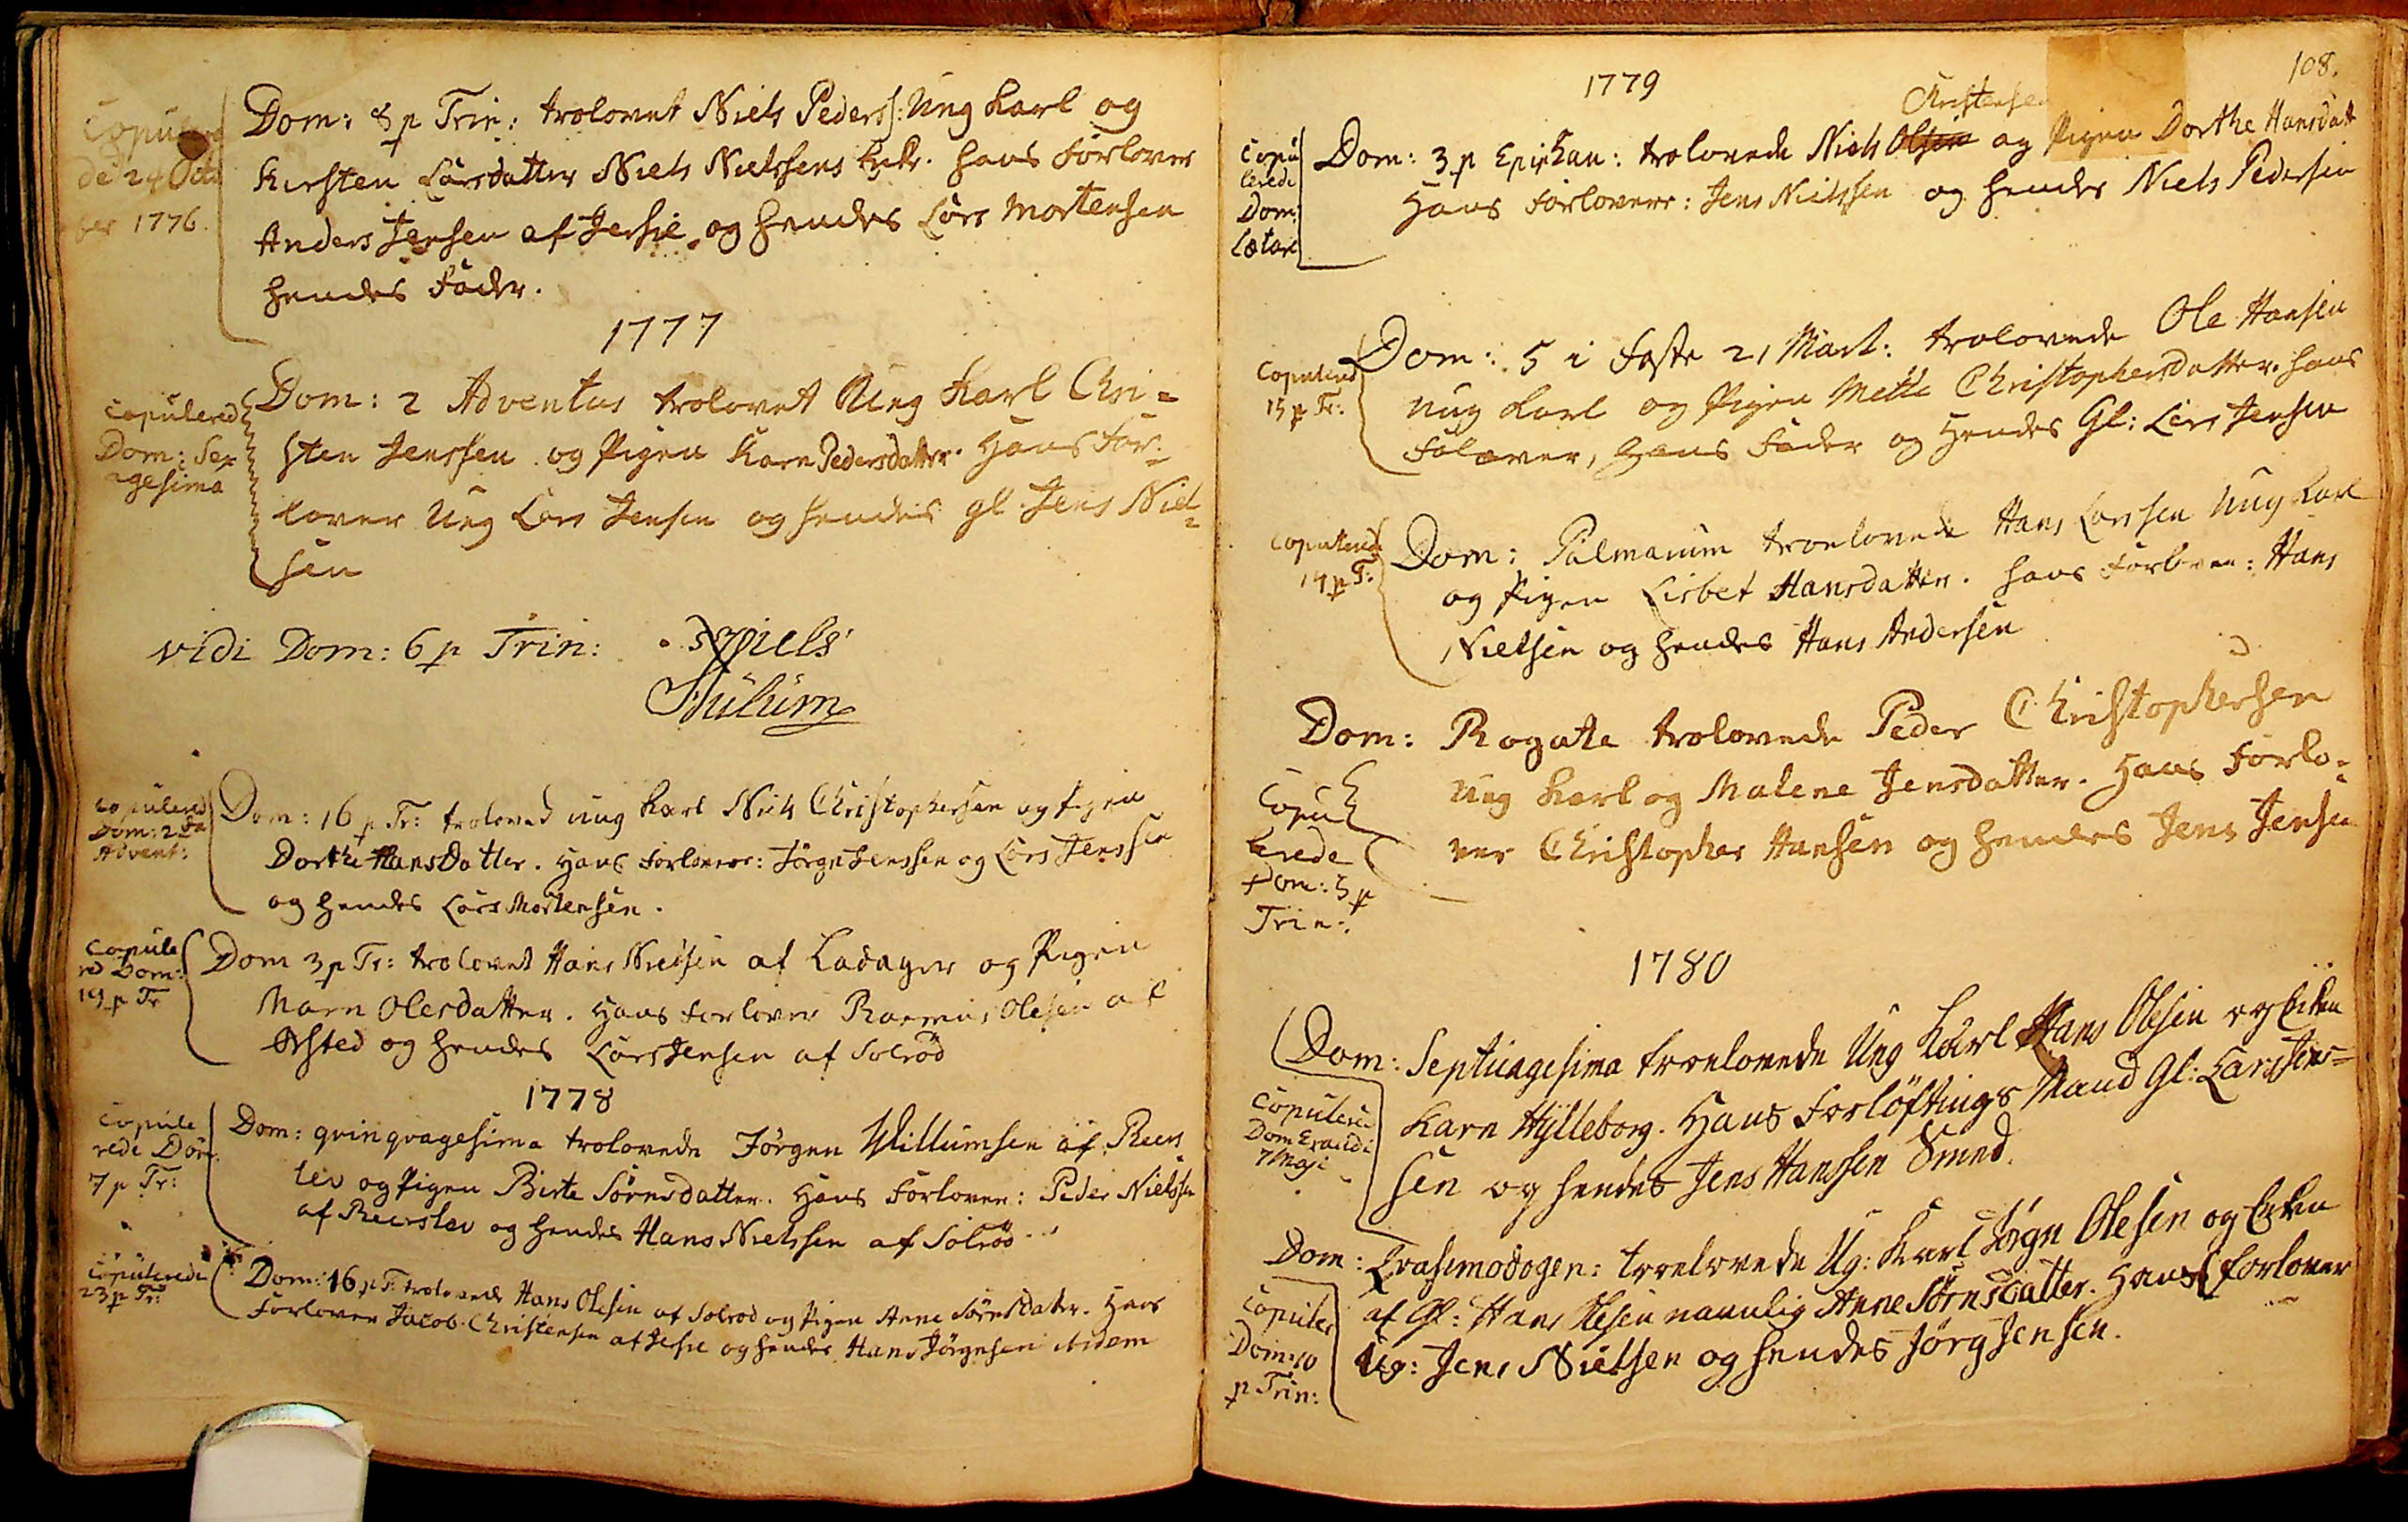Dom: 8 p Trin: trolovet Niels Pederss: Ung karl og
Kirsten Larsdatter Niels Nielsens Enke. hans Forlover
Anders Jensen af Jersie og hendes Lars Mortensen
hendes Fader.
Copulere
de 24 Octo
ber 1776.  
1777
Dom: 2 Adventus trolovet Ung karl Chri¬
sten Jenssen og Pigen Karn Pedersdatter. Hans For¬
lover Ung Lars Jensen og hendes gl. Jens Niel¬
sen
Copulered
Dom: Sex
agesima
Vidi Dom: 6 p Trin: Niels
Aulum
Dom: 16 p Tr: trolovet ung karl Niels Christophersen og Pigen
Dorthe HansDatter. Hans Forlovere: Jørgn Jenssen og Lars Jenssen
og hendes Lars Mortensen.
Copulered
Dom: 2da
Advent:
Dom 3 p tr: trolovet Hans Nielsen af Ladager og Pigen
Marn Olesdatter. Hans Forlover Rasmus Olesen af
Ørsted og hendes Lars Jensen af Solrød
Copule
red Dom:
19 p Tr.
1778
Dom: qvinqvagesima trolovede Jørgen Willumsen af Reers¬
lev og Pigen Birte Sørnsdatter. Hans Forlover: Peder Nielssen
af Reerslev og hendes Hans Nielssen af Solrød
Copule
rede Dom.
7 p Tr:      
Dom: 16 p T: trolovede Hans Olesen af Solrød og Pigen Anne Sørnsdatter. Hans
Forlover Jacob Christensen af Jersie og hendes Hans Jørnsen ibidem
Copulerede
23 p Tr:        
108.
1779
Christensen
Dom: 3 p Epiphan: trolovede Niels Olsen og Pigen Dorthe Hansdatt
Hans Forlovere: Jens Nielssen og hendes Niels Pedersen
Copu
lerede
Dom:
Lætar
Dom: 5 i Faste 21 Mart: trolovede Ole Hansen
ung karl og Pigen Mette Christophersdatter. hans
Forlover, hans Fader og Hendes Gl: Lars Jensen
Copulered
15 p Tr:
Dom: Palmarum troelovede Hans Larssen Ungkarl
og Pigen Lisbet Hansdatter. hans Forlover: Hans
Nielsen og hendes Hans Andersen
Copulered
14 p T:
Dom: Rogate trolovede Peder Christophersen
Ung karl og Malene Jensdatter. Hans Forlo¬
var Christopher Hansen og hendes Jens Jensen
Copu
lerede
Dom: 5 p
Trin:
1780
Dom: Septuagesima troelovede Ung Karl Hans Olesen og Enken
Karn Hylleborg. Hans Forløftings Mand Gl: Lars Jens¬
sen og hendes Jens Hanssen Smed.
Copulered
Dom Exaudi
7 Maji
Dom: Qvasimodogen: troelovede Un: Karl Jørgn Olesen og Enken
af Gl: Hans Olesen navnlig Anne Sørnsdatter. Hans Forlover
Ug: Jens Nielsen og hendes Jørg Jensen.
Copuler
Dom: 10
p Trin:
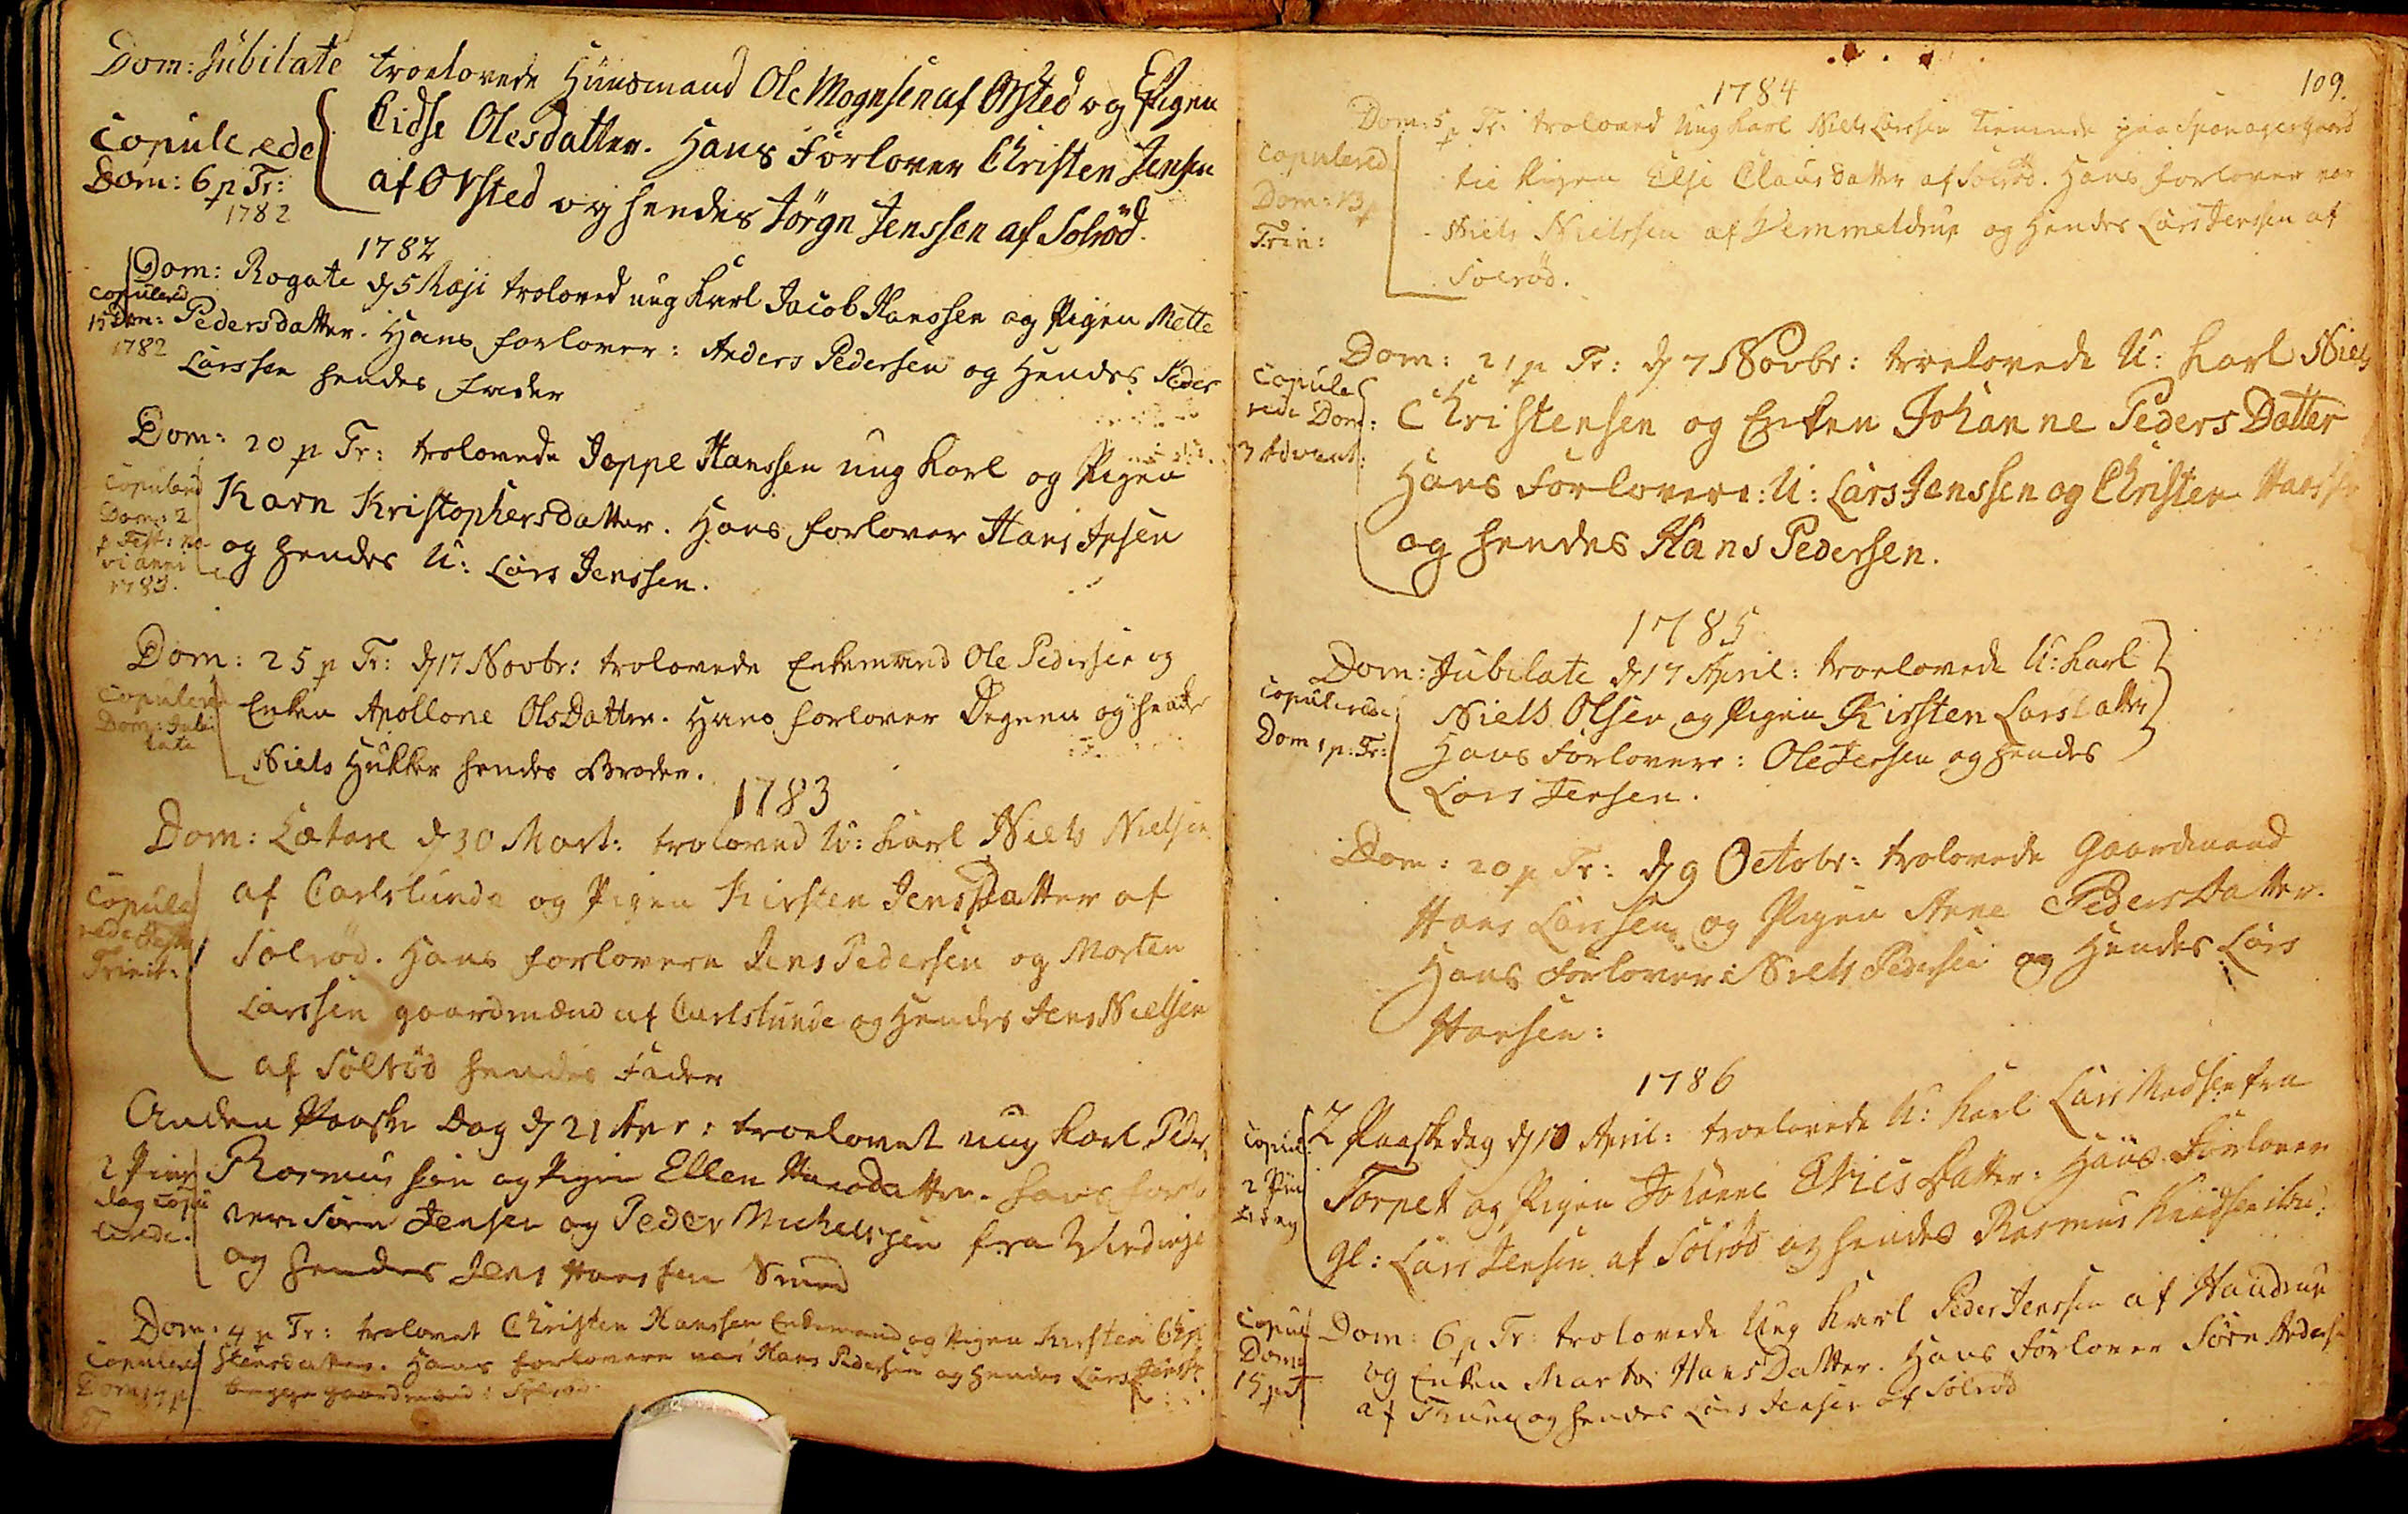Dom: Jubilate troelovede Huusmand Ole Mognsen af Ørsted og Pigen
Cidse Olesdatter. Hans Forlover Christen Jensen
af Ørsted og hendes Jørgn Jenssen af Solrød.
Copulerede
Dom: 6 p Tr:
1782
1782
Dom: Rogate d 5 Maji troloved ung karl Jacob Hanssen og Pigen Mette
Pedersdatter. Hans Forlover: Anders Pedersen og Hendes Peder
Larssen hendes Fader
Copulered
15 Dom:
1782
Dom: 20 p Tr: trolovede Jeppe Hanssen ung karl og Pigen
Karn Kristophersdatter. Hans Forlover Hans Ipsen
og hendes U: Lars Jenssen.
Copulered
Dom: 2
p Fest: No¬
vi Anni
1783.
Dom: 25 p Tr: d 17 Novbr: trolovede Enkemand Ole Pedersen og
Enke Apollone OlsDatter. Hans Forlover Degnen og hendes
Niels Hukker hendes Broder.
Copule
Dom: Jubi  
late
1783
Dom: Lætare d 30 Mart: troloved U: karl Niels Nielsen
af Carlslunde og Pigen Kirsten JensDatter af
Solrød. Hans Forlovere Jens Pedersen og Morten
Larssen gaardmænd af Carlslunde og Hendes Jens Nielsen
af Solrød hendes Fader
Copule
rede Fest
Trinit:    
Anden Paaske Dag d 21 Apr: troelovet ung karl Peder
Rasmussen og Pigen Ellen Hansdatter. hans for
lover Sørn Jensen og Peder Michelssen fra Windinge
og hendes Jens Hanssen Smed
2 Pinz
dag Copu   
lerede.
Dom: 4 p Tr: trolovet Christen Hanssen Enkemand og Pigen Kirsten Chri
stensdatter. Hans forlovere var Hans Pedersen og hendes Lars Jenssen
begge Gaardmænd i Solrød.
Copulered
Dom: 14 p   
Tr
109.
1784
Dom: 5 p Tr: troloved Ung karl Niels Larssen Tienende paa Spanagergaard
til Pigen Else Clausdatter af Solrød. Hans forlover var
Niels Nielssen af Vemmeldrup og Hendes Lars Jenssen af
Solrød.
Copulered
Dom: 13 p     
Trin:
Dom: 21 p Tr: d 7 Novbr: troelovede U: karl Niels
Christensen og Enken Johanne Peders Datter
Hans Forlovere: U: Lars Jenssen og Christen Hanssen
og hendes Hans Pedersen.
Copule
rede Dom:     
3 Advent:
1785
Dom: Jubilate d 17 April: troelovede U:karl
Niels Olsen og Pigen Kirsten LarsDatter
Hans Forlovere. Ole Jensen og hendes
Lars Jensen.
Copulerede
Dom 1 p: Tr:
Dom: 20 p Tr: d 9 Octobr: trolovede Gaardmand
Hans Larssen og Pigen Anne PedersDatter.
Hans Forlover: Niels Pedersen og Hendes Lars
Hansen:
1786
2 Paaskedag d 17 April: troelovede U: karl Lars Madsen fra
Torpet og Pigen Johanne EricsDatter: Hans Forlover
gl: Lars Jensen af Solrød og hendes Rasmus Knudsen ibm:
Copul
2 Pin
zedag
Dom: 6 p Tr: trolovede Ung Karl Peder Jenssen af Haudrup
og Enken Marta HansDatter. Hans Forlover Sørn Andersen
af Thune og hendes Lars Jensen af Solrød
Copul
Dom:
15 p T